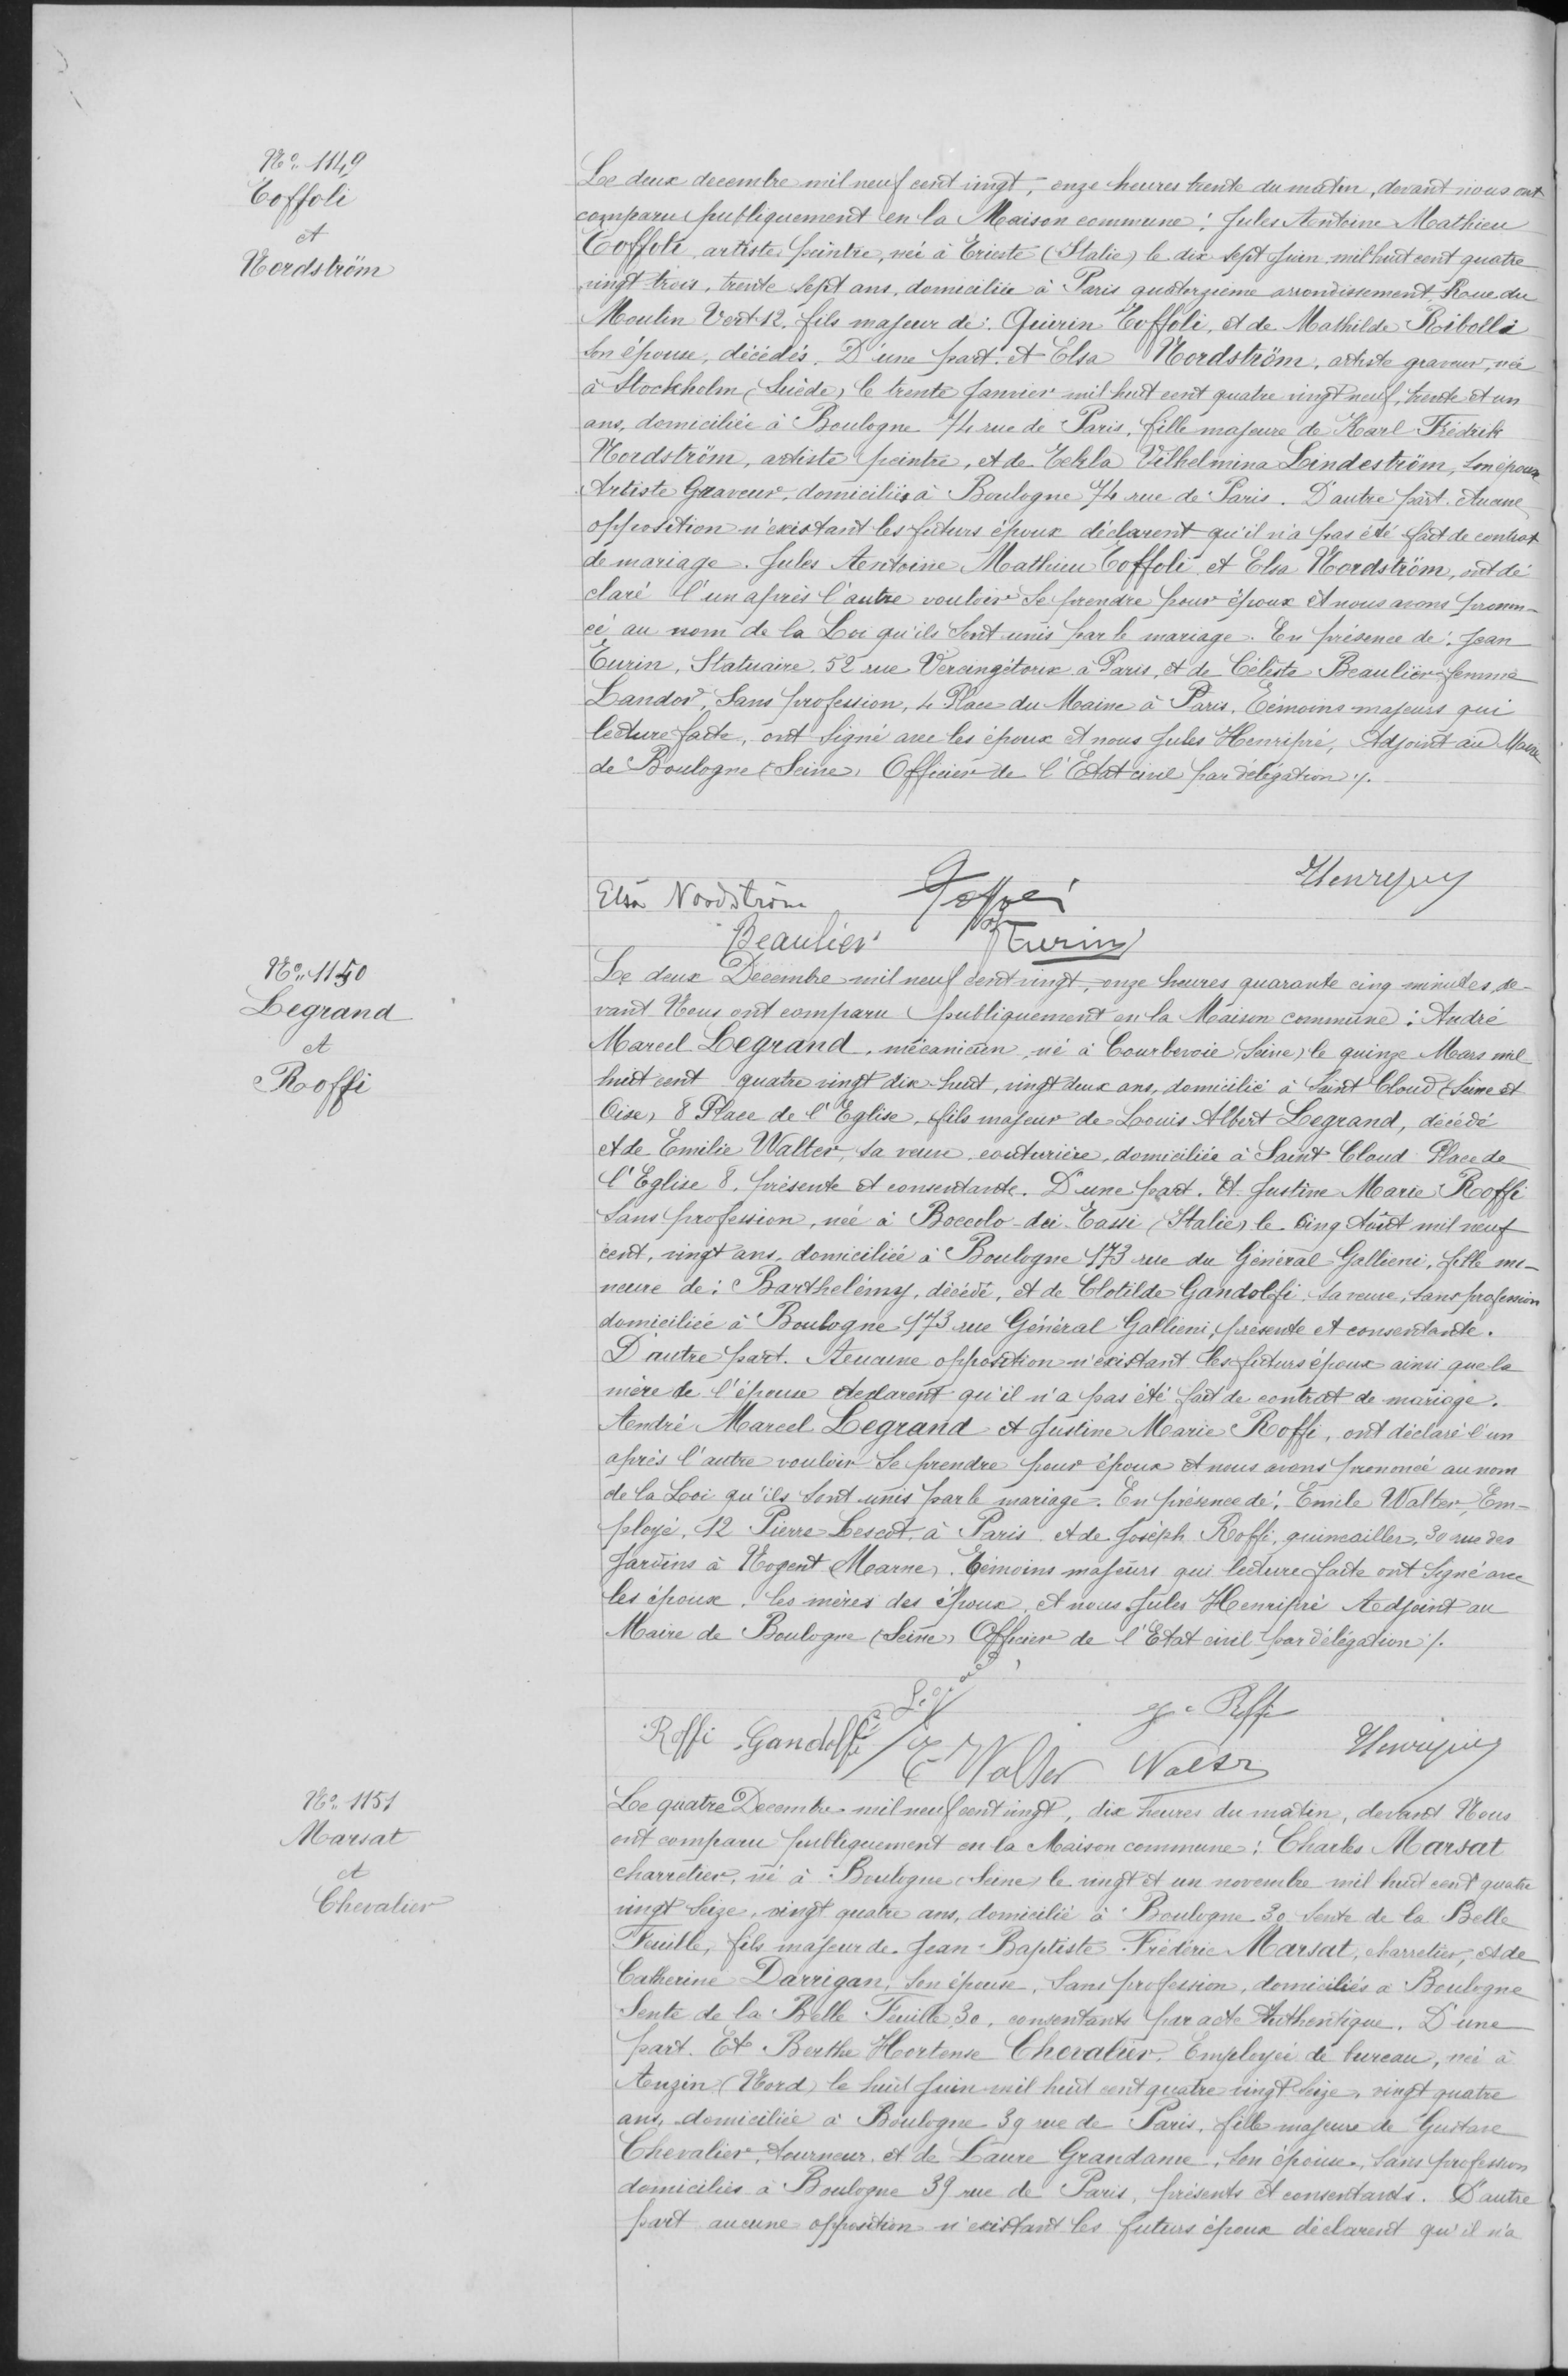N°1149
Toffoli
et
Nordström
Le deux décembre mil neuf cent vingt, onze heures huit du matin, devant nous ont
comparu publiquement en la Maison commune: Julien Antoine Mathieu
Toffoli, artiste peintre, né à Erieste (Italie) le dix-sept juin mil huit cent quatre
vingt-trois, trente sept ans, domiciliée à Paris quatorzième arrondissement Rue du
Moulin Vert 12. fils majeur de: Quirin Toffoli, et de Mathilde Ribolli
son épouse, décédés. D'une part. Et Elsa Nordström, artiste graveur, née
à Stockholm (Suède) le trente janvier mil huit cent quatre vingt neuf, tente et un
ans, domiciliée à Boulogne 74 rue de Paris, fille majeure de Karl Frédrik
Nordström, artiste peintre, et de Ieklka Vilhelmina Lindeström, son épouxe
artiste graveur, domicilié à Boulogne 74 rue de Paris. D'autre part. Aucune
opposition n'existant les futurs époux déclarent qu'il n'a pas été fait de contrat
de mariage. Jules Antoine Mathieu Toffolé et Elsa Nordström, ont dé
claré l'un après l'autre vouloir se prendre pour époux et nous avons pronon-
cé au nom de la Loi qu'ils sont unis par le mariage. En présence de: Joan
Turin, statuaire, 52, rue Vercingétorix à Paris, et de Céleste Beualieu femme
Landov sans profession, 4 Place du Maine à Paris, témoins majeurs qui
lecture faite, ont signé avec les époux et nous Jules Henripré, Adjoint au Maire
de Boulogne (Seine) Officier de l'Etat civil par délégation ./.
N°1140
Legrand
et
Roffi
Le deux Décembre mil neuf cent vingt, onze heures quarante cinq minutes de-
vant Nous ont comparu publiquement en la Maison commune: André
Marcel Legrand, mécanicien, né à Courbevoie (Seine) le quinze Mars mil
huit cent quatre vingt dix-huit, vingt deux ans, domicilié à Saint Cloud (Seine et
Oise) 8 Place de l'Eglise, fils majeur de Louis Albert Legrand, décéé
et de Emilie Walter, sa veuve, couturière, domiciliée à Saint Cloud Place de
l'Eglise 8, présente et consentante. D'une part. Et Justine Marie Roffi
sans profession, née à Boccolo-dei-Cassi (Italie) le cinq Août mil neuf
cent, vingt ans, domiciliée à Boulogne 173 rue du Général Gallieni, fille ma-
jeure de: Barthélémy, décédé, et de Clotilde Gandolfi, sa veuve, sans profession
domiciliée à Boulogne 173 rue Général Gallieni, présente et consentante.
D'autre part. Aucune opposition n'existant les futurs époux ainsi que la
mère de l'épouse déclarent qu'il n'a pas été fait de contrat de mariage.
André Marcel Legrand et Justine Marie Roffi, ont déclaré l'un
après l'autre vouloir se prendre pour époux et nous avons prononcé au nom
de la Loi qu'ils sont unis par le mariage. En présence de: Emile alter, Em-
ployé, 12 Pierre Cescot, à Paris et de Joseph Roffi, quincailler, 30 rue des
Jardins à Nogent (Marne) témoins majeurs qui lecture faite ont signé avec
les époux, les mères des époux et nous Jules Hennipré Adjoint au
Maire de Boulogne (Seine) Officier de l'Etat civil, par délégation ./.
N° 1151
Marsat
et
Chevalier
Le quatre Décembre mil neuf cent vingt, dix heures du matin, devant Nous
ont comparu publiquement en la Maison commune: Charles Marsat
charretier, né à Boulogne (Seine) le vingt et un novembre mil huit cent quatre
vingt seize, vingt quatre ans, domicilié à Boulogne 30 sente de la Belle
Feuille, fils majeur de Jean-Baptiste-Frédéric Marsat, charretier, et de
Catherine Darrigan, son épouse, sans profession, domiciliés à Boulogne
sente de la belle Feuille 30, consentant par acte authentique. D'une
part. Et Berthe Hortense Chevalier. Employé de bureau, née à
Auzin (Nord) le huit juin mil huit cent quatre vingt seize, vingt quatre
ans, domiciliée à Boulogne 39 rue de Paris, fille majeure de Gustave
Chevalier, tourneur, et de Laure Grandame, son épouse, sans profession
domiciliés à Boulogne 39 rue de Paris, présents et consentants. D'autre
part aucune opposition n'existant les futurs époux déclarent qu'il n'a
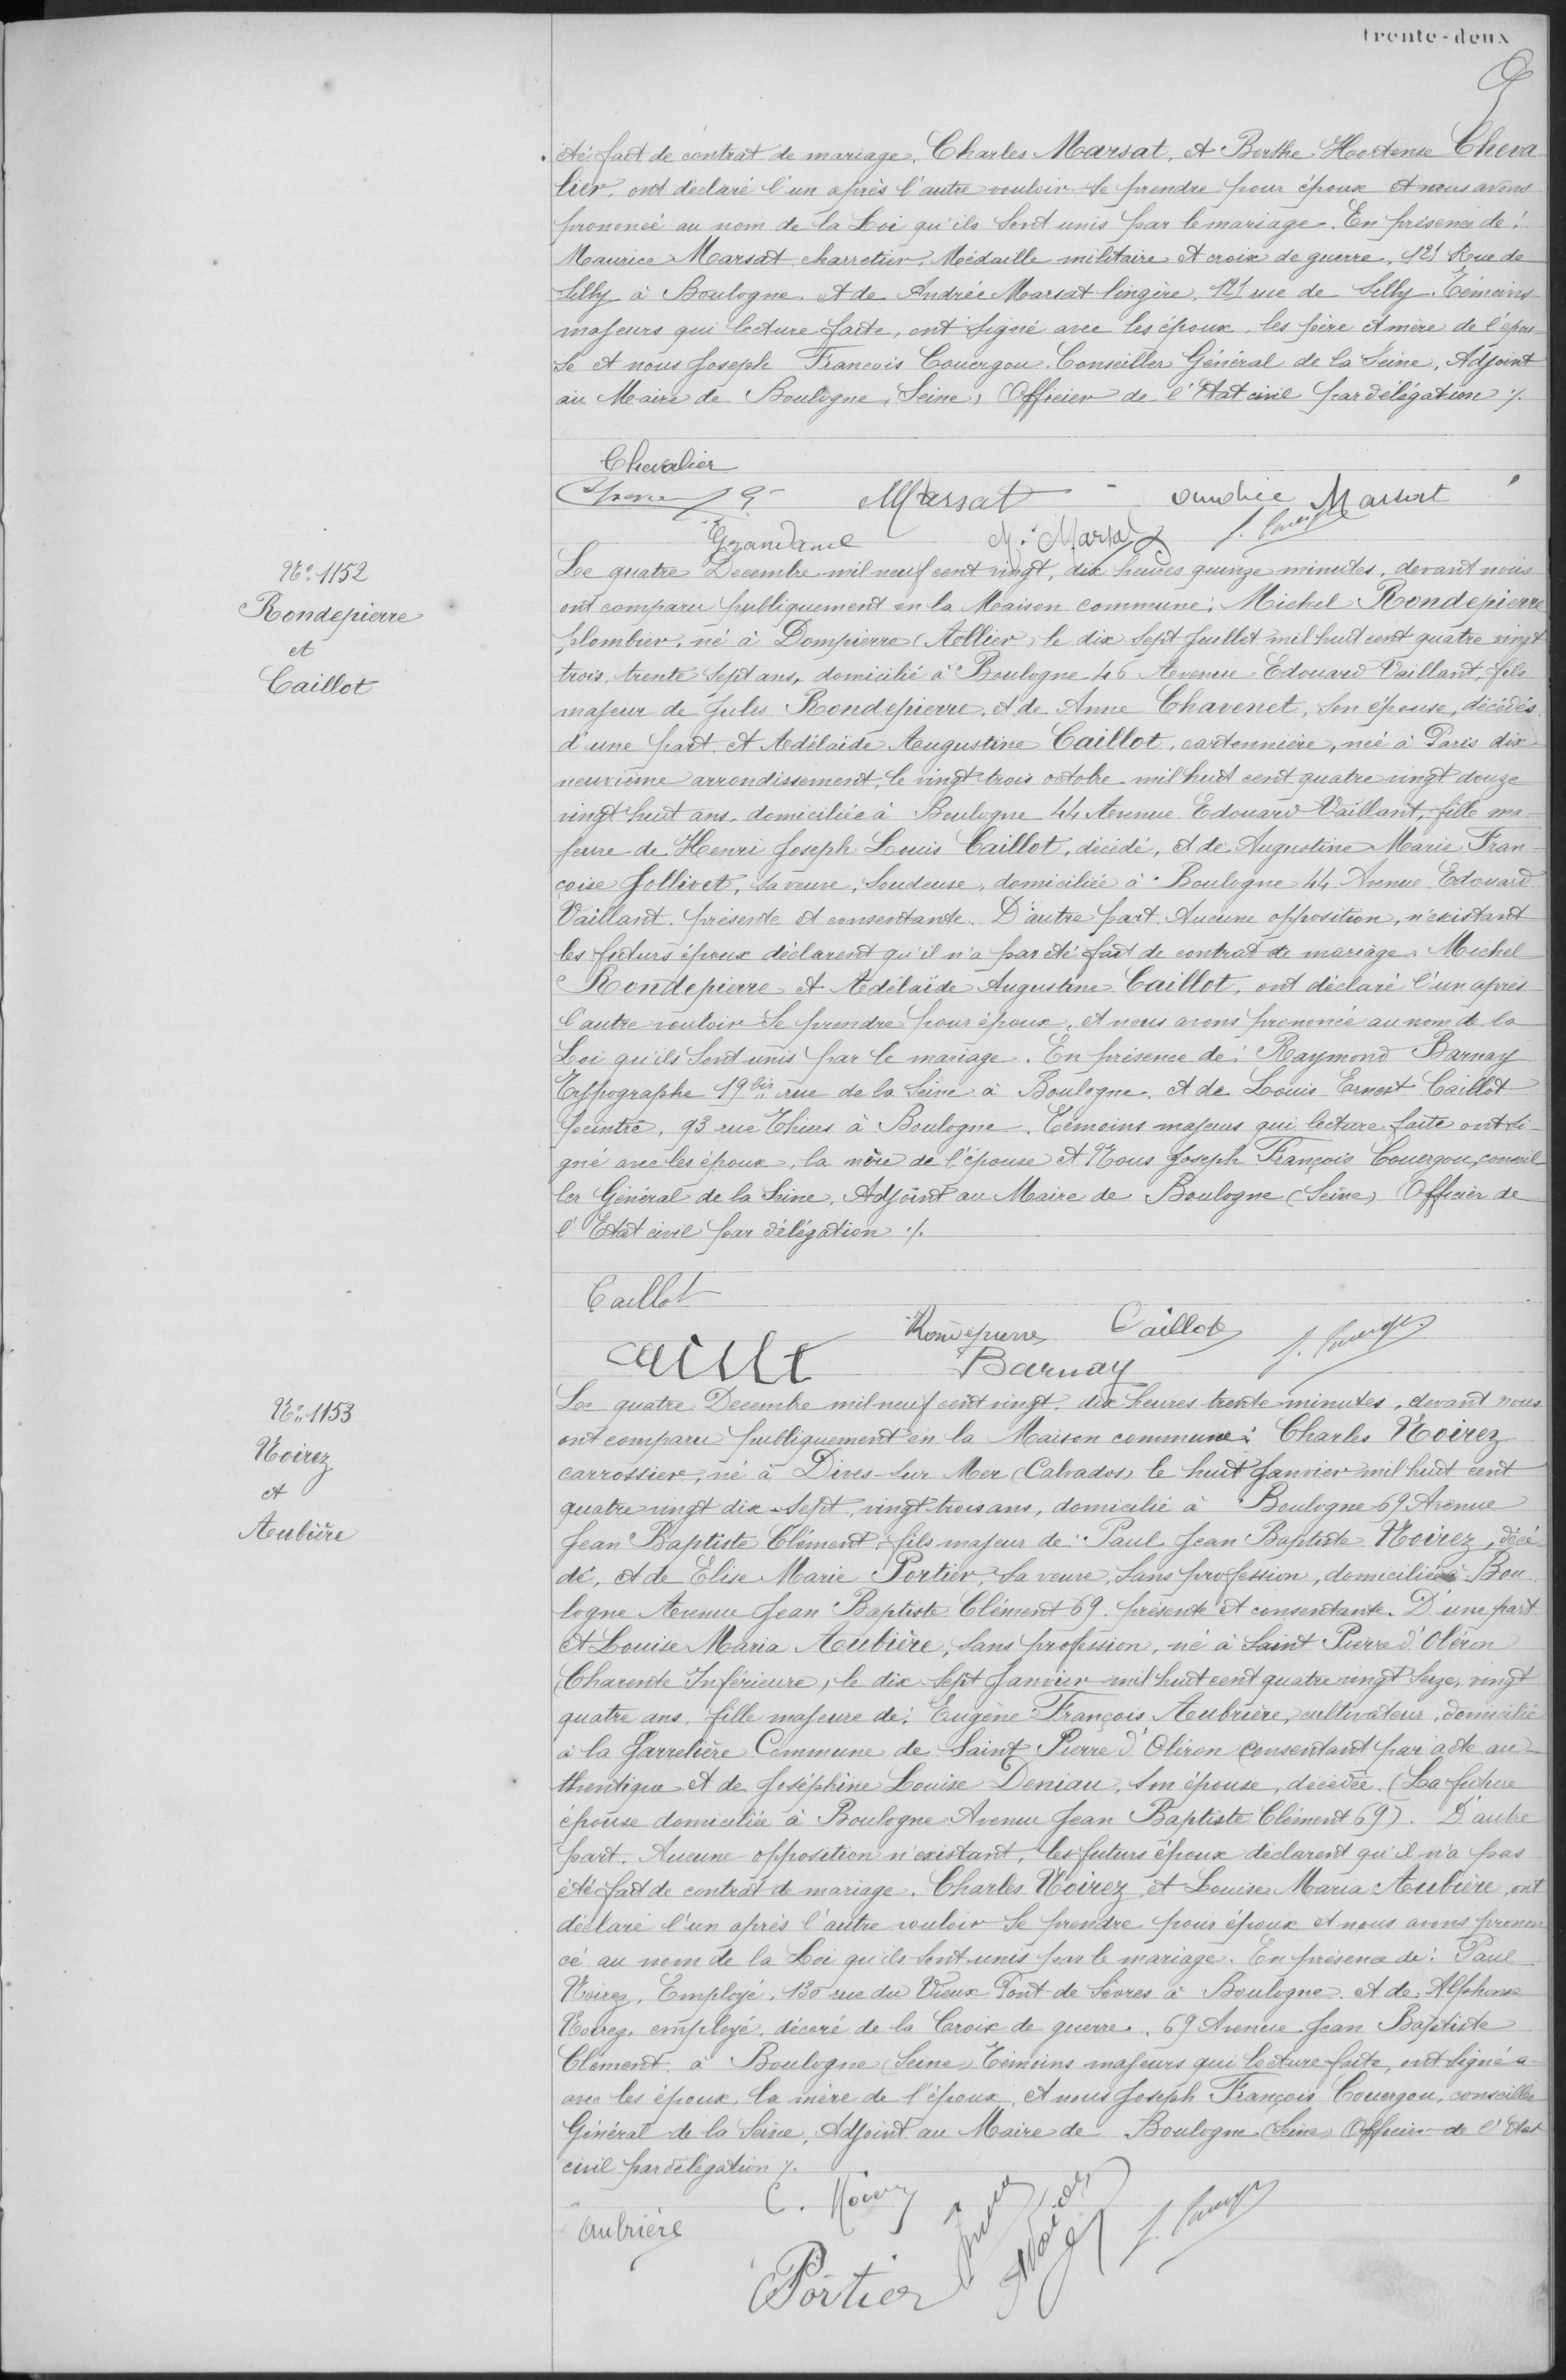été fait de contrat de mariage, Charles Marsat, et Berthe Hortense Cheva
lier, ont déclaré l'un après l'autre vouloir se prendre pour époux et nous avons
prononcé au nom de la Loi qu'ils sont unis par le mariage. En présence de:
Maurice Marsat charretier, médaille militaire et croix de guerre, 121 Rue de
Silby à Boulogne et de André Marsat lingère, 121 rue de Silby. Témoins
majeurs qui lecture faite, ont signé avec les époux, les père et mère de l'épou
se et nous Joseph François Couergou. Conseiller Général de la Seine, Adjoint
au Maire de Boulogne, Seine, Officier de l'Etat civil par délégation ./.
N°1152
Rondepierre
et
Caillot
Le quatre Décembre mil neuf cent vingt, dix heures quinze minutes, devant nous
ont comparu publiquement en la Maison commune: Michel Rondepierre
plombier, né à Dompierre (Allier) le dix sept juillet mil huit cent quatre vingt
trois, trente sept an, domicilié à Boulogne 46 Avenue Edouard Vaillant, fils
majeur de Jules Rondepierre, et de Anne Chavenet, son épouse, décédés
d'une part et Adélaïde Augustine Caillot, cartonnière, née à Paris dix-
neuvième arrondissement, le vingt trois octobre mil huit cent quatre-vingt douze
vingt huit ans, domicilié à Boulogne 44 Avenue Edouard Vaillant, fille ma-
jeure de Henri Joseph Louis Caillot, décédé, et de Augustine Marie Fran-
çoise Jollivet, sa veuve, soudeuse, domiciliée à Boulogne 44 Avenue Edouard
Vaillant, présents et consentants. D'autre part. Aucune opposition n'existant
les futurs époux déclarent qu'il n'a pas été fait de contrat de mariage. Michel
Rondepierre et Adélaïde Augustine Caillot, ont déclaré l'un après
l'autre vouloir se prendre pour époux, et nous avons prononcé au nom de la
Loi qu'ils sont unis par le mariage. En présnce de: Raymond Barnay
typographe 19bis rue de la Seine à Boulogne et de Louis Ernest Caillot
Yocintre, 93 rue Thiers à Boulogne. Témoins majeurs qui lecture faite ont si-
gné avec les époux, la mère de l'épouse et Nous Joseph François Couergou, conseil
ler Général de la seine, Adjoint a Maire de Boulogne (Seine) Officier de
l'Etat civil par délégation ./.
N°1153
Noirez
et
Aubière
Le quatre Décembre mil neuf cent vingt dix heures trente minutes, devant nous
ont comparu publiquement en la Maison commune: Charles Noirez
carrossier, né à Dives-sur Mer (Calvados) le huit Janvier mil huit cent
quatre vingt dix-sept, vingt-trois ans, domicilié à Boulogne 69 Avenue
Jean Baptiste Clément fils majeur de Paul Jean Baptiste Noirez, décé-
dé, et de Elise Marie Portier, sa veuve, sans profession, domiciliée à Bou
logne Avenue Jean Baptiste Clément 69 présente et consentante. D'une part
et Louie MAria Aubière, sans profession, né à Saint Pierre d'Oléron
(Charente Inférieure), le dix sept Janvier mil huit cent quatre vingt treize vingt
quatre ans, fille majeur de: Eugène François Aubrière, cultivateur, domicilié
à la Garrelière Commune de Saint Pierre d'oléron consentant par acte au-
thentique et de Joséphine Louise Deniau, son épouse, décédée. (La future
épouse domiciliée à Boulogne Avenue Jean Baptiste Clément 69). D'autre
part. Aucune opposition n'existant, les futurs époux déclarent qu'il n'a pas
été fait de contrat de mariage. Charles Noirez et Louise Maria Aubière ont
déclaré l'un après l'autre vouloir se prendre pour époux et nous avons pronon
cé au nom de la Loi qu'ils sont unis par le mariage. En présence de: Paul
Noirez, Employé, 130 rue du Vieux Pont de Sèvres à Boulogne et de Alphonse
Vobrez, employé, décoré de la Croix de guerre 69 Avenue Jean Baptiste
Clément à Boulogne (Seine) Témoins majeurs qui lecture faite, ont signé a-
avec les époux la mère de l'époux, et nous Joseph François Couergou, conseiller
Général de la Seine, Adjoint au Maire de Boulogne (Seine) Officier de l'Etat
civil par délégation ./.